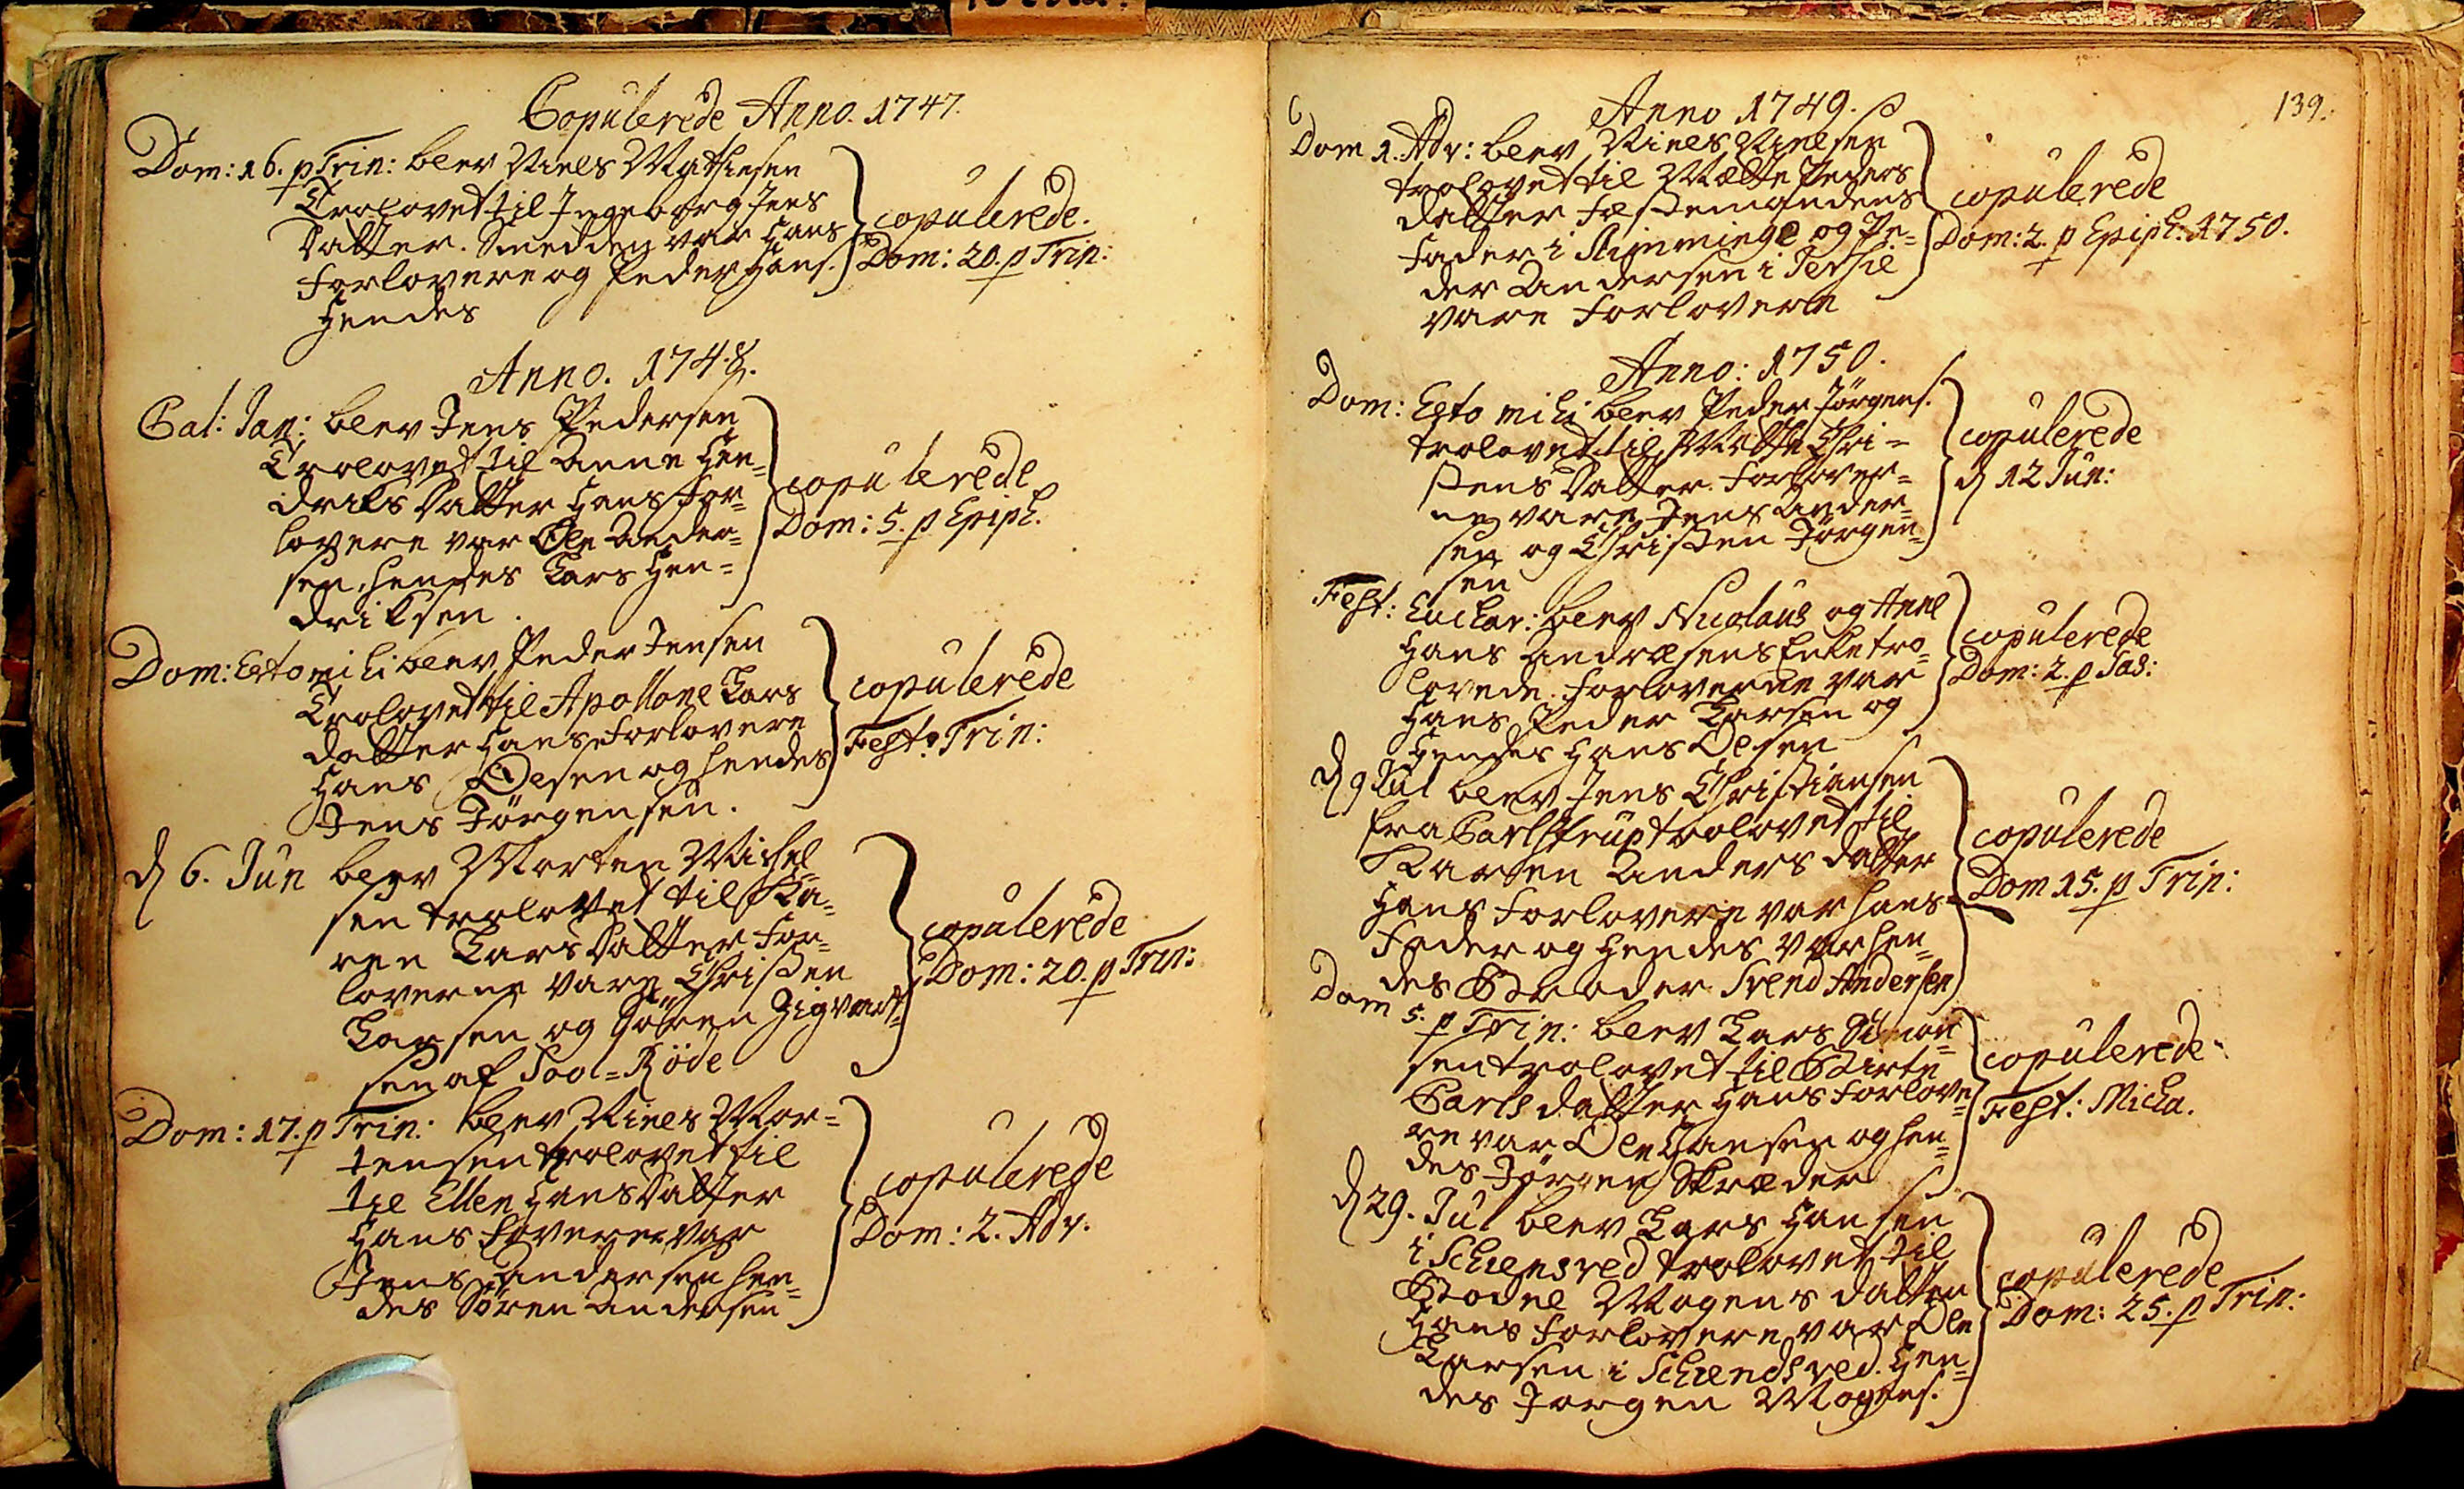Copulerede Anno. 1747.
Dom: 16. p Trin: blev Niels Mathiesen
Trolovet til Ingeborg Jens
Datter. Smedden var Hans
Forlovere og Peder Hans.
Hendes
Copulerede
Dom: 20. p Trin:
Anno. 1748.
Cal: Jan: blev Jens Pedersen
Trolovet til Anne Hen¬
driks Datter Hans For¬
lovere var Ole Ander¬
sen, hendes Lars Hen¬
driksen.
Copulerede
Dom: 5. p Epiph.
Dom: Esto mihi blev Peder Jensen
Trolovet til Apollone Lars
datter Hans Forlovere
Hans Olsen og hendes
Jens Jørgensen.
Copulerede
Fest: Trin:
d 6. Jun blev Morten Michel¬
sen trolovet til Ka¬
ren Lars Datter For¬
loverne vare Christen
Larsen og Søren Zigvardt¬
sen af Sool-Røde
Copulerede
Dom: 20. p Trin
Dom: 17. p Trin: blev Niels Mor¬
tensen trolovet til
til Ellen Hans Datter
Dom: 2. Adv.
Hans Fovere## var
Jens Andersen hen¬
des Søren Andersen
Copulerede
139.
Anno 1749.
Dom 1. Adv: blev Niels Nielsen
trolovet til Mætte Peders
datter Fæstemandens
Fader i Slimminge og Pe¬
der Andersen i Jersie
vare Forlovere
Copulerede
Dom: 2. p Epiph. 1750.
Anno: 1750.
Dom: Esto Mihi blev Peder Jørgens.
trolovet til Mætte Chri¬
stens Datter. Forlover¬
ne vare Jens Ander¬
sen og Christen Jørgen¬
sen
Copulerede
d 12 Jun:
Fest: Euc Ear:## blev Nicolaus og Anne
Hans Andræsens Enke tro¬
lovede. Forloverne var
Hans Peder Larsen og
Hendes Hans Olsen
Copulerede
Dom: 2. p Pas:
d 9 Jul blev Jens Christiansen
fra Carlstrup trolovet til
Karen Anders datter
Hans Forlovere var hans
Fader og hendes var hen¬
des Broder Svend Andersen
Copulerede
Dom 15. p Trin:
Dom 5. Trin: blev Lars Simon¬
sen trolovet til Birte
Carlsdatter Hans Forlove¬
re var Ole Hansen og hen¬
des Jørgen Skræder
Copulerede
Fest: Micha.
d 29. Jul blev Lars Hansen
i Sckiensved trolovet til
Bodel Mogensdatter
Hans Forlovere var Ole
Larsen i Sckiendsved. Hen¬
des Jørgen Mogens.
Copulerede
Dom: 25. p Trin:
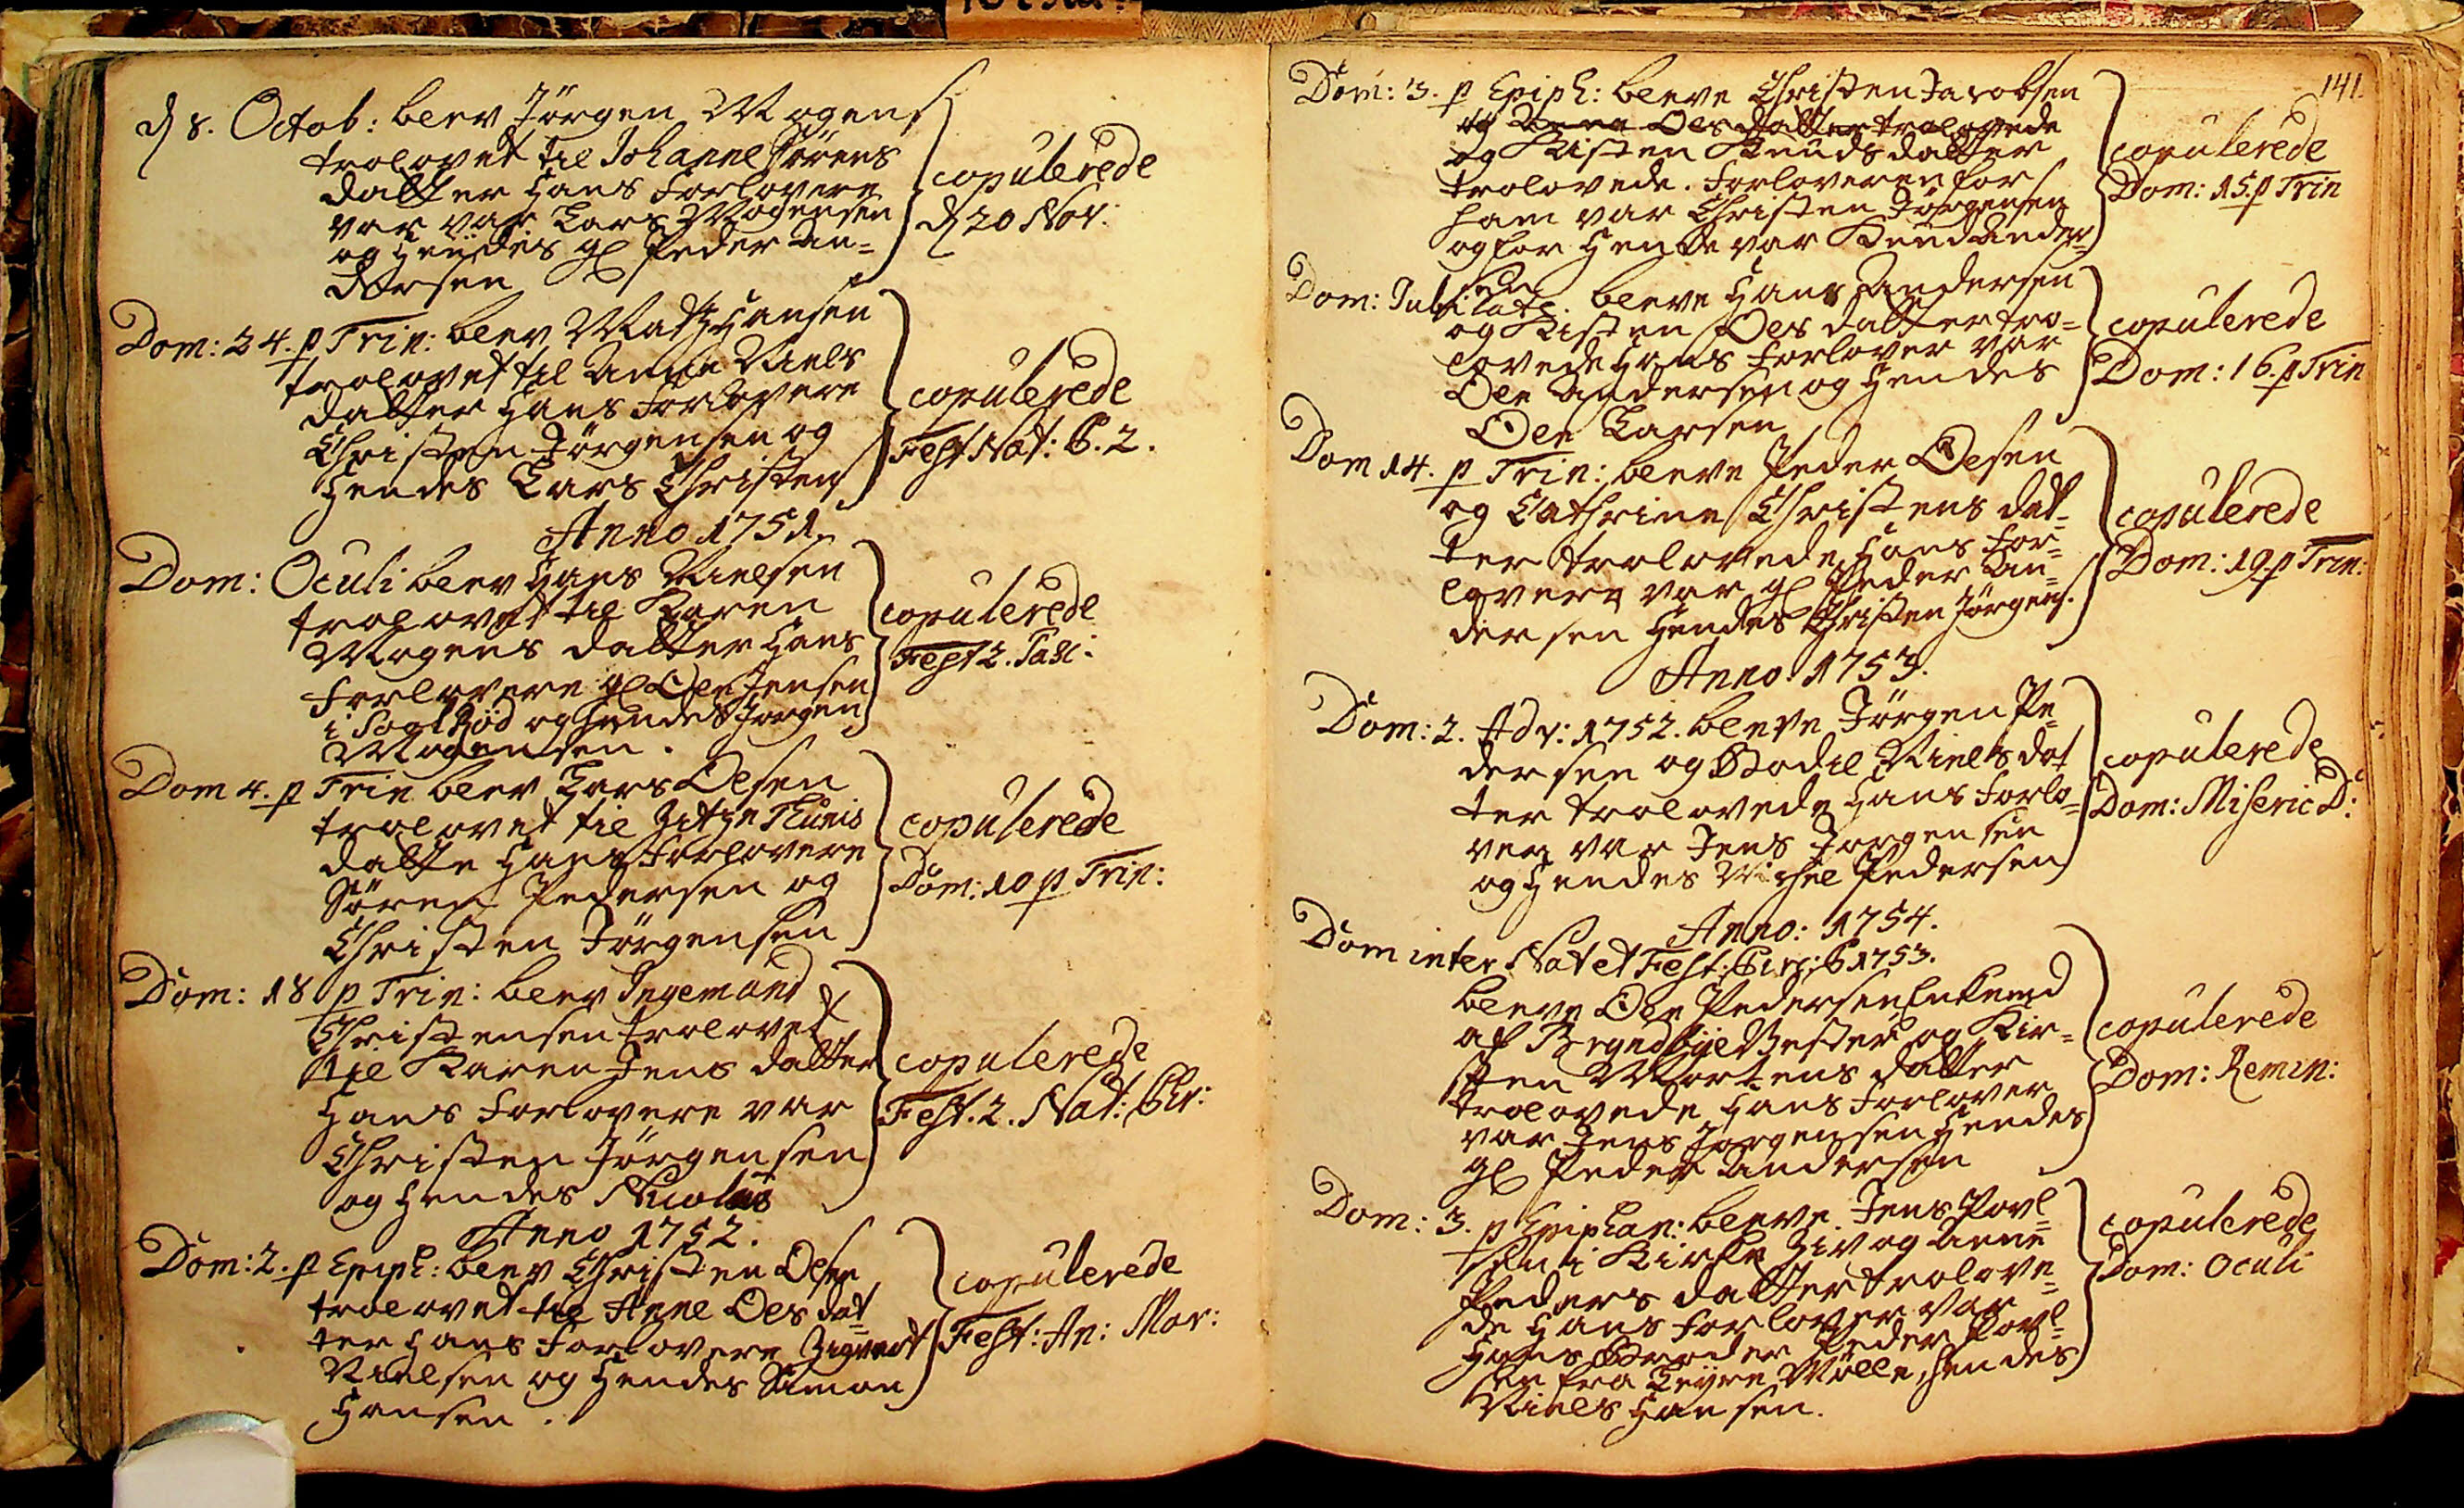d 8. Octob: blev Jørgen Mogens
trolovet til Johanne Sørens
datter Hans Forlovere
var var Lars Mogensen
og hendes gl Peder An¬
dersen
Copulerede
d 20 Nov:
Dom: 24. p Trin: blev Matz Hansen
trolovet til Anne Niels
datter Hans Forlovere
Christen Jørgensen og
Hendes Lars Christens
Copulerede
Fest Nat: C.2.
Anno 1751.
Dom: Oculi blev Hans Nielsen
Trolovet til Karen
Mogens datter Hans
Forlovere gl Ole Jensen
i SoolRød og hendes Jorgen
Mogensen.
Copulerede
Fest 2. Pasc:
Dom 4. p Trin blev Lars Olsen
trolovet til Zitze Thunis
datter Hans Forlovere
Søren Pedersen og
Christen Jørgensen
Copulerede
Dom: 10 p Trin:
Dom: 18 p Trin: blev Ingemand
Christensen trolovet
til Karen Jens datter
Hans Forlovere var
Christen Jørgensen
og hendes Nicolus
Copulerede
Fest. 2. Nat: Chr:
Anno 1752.
Dom: 2. p Epiph: blev Christen Olsen
trolovet til Anne Ols dat¬
ter Hans Forlovere Zigvart
Nielsen og Hendes Simon
Hansen.
Copulerede
Fest: An: Mar:
141.
Dom: 3. p Epiph: bleve Christen Jacobsen
og Anna Olsdatter trolovede
og Kirsten Knudsdatter
trolovede. Forloveren for
ham var Christen Jørgensen
og for Hende var Knud Ander¬
sen.
Copulerede
Dom: 15. p Trin
Dom: Jubilate. bleve Hans Andersen
og Kirsten Ols datter tro¬
lovede Hans Forlover var
Ole Andersen og Hendes
Ole Larsen
Copulerede
Dom: 16. p Trin
Dom 14. p Trin: bleve Peder Olsen
og Cathrine Christens dat¬
ter trolovede, Hans For¬
lovere var gl Peder An¬
dersen Hendes Christen Jørgens.
Copulerede
Dom: 19. p Trin:
Anno: 1753.
Dom: 2. Adv: 1752. bleve Jørgen Pe¬
dersen og Bodel Nielsdat
ter trolovede Hans Forlo¬
ver var Jens Jørgensen
og Hendes Michel Pedersen
Copulerede
Dom: Miseric D:
Anno: 1754.
Dom inter Nat et Fest: Circ: C 1753.
bleve Ole Pedersen Enkemd
af Bryndbye Vester og Kir¬
sten Mortens datter
trolovede Hans Forlover
var Jens Jørgensen Hendes
gl Peder Andersen
Copulerede
Dom: Remin:
Dom: 3. p Epiphan: bleve Jens Povl¬
sen i Kirke Ziv og Anne
Peders datter trolove¬
de Hans Forlover var
Hans Broder Peder Povl¬
sen fra Leyre Mülle, hendes
Niels Hansen.
Copulerede
Dom: Oculi